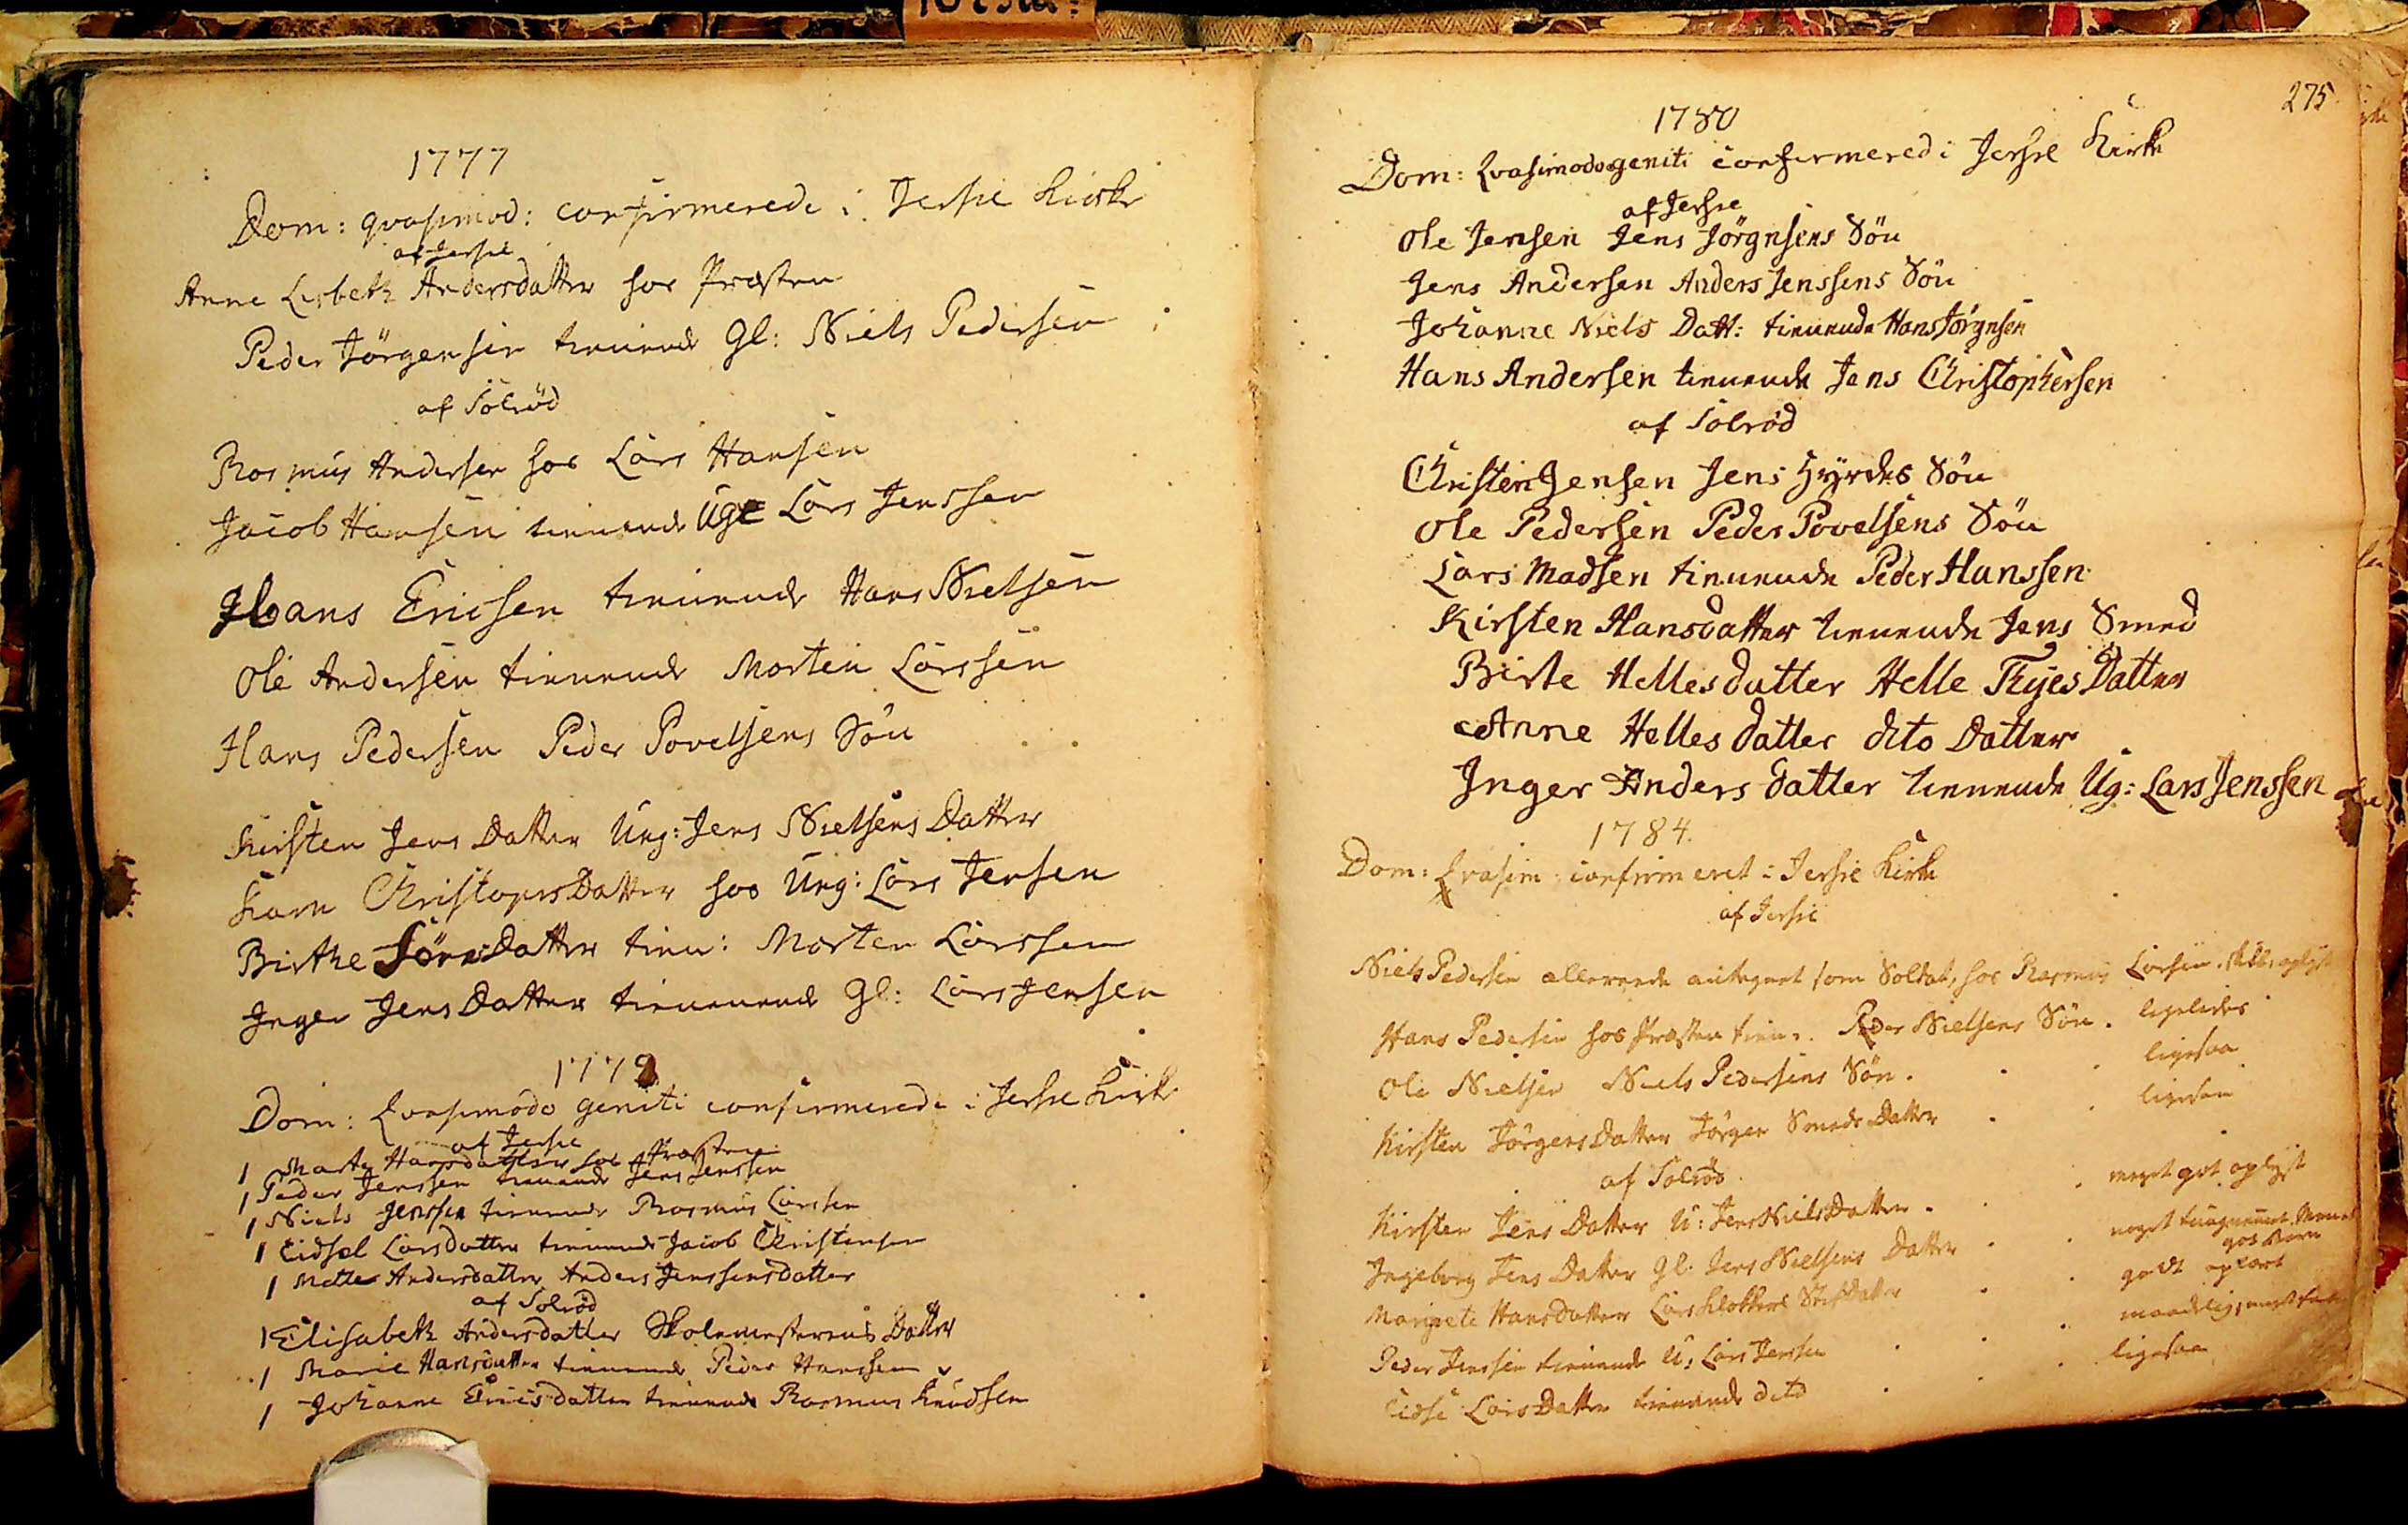1777
Dom: Qvasimod: Confirmerede i Jersie Kirke
af Jersie
Anne Lisbeth Andersdatter hos Præsten
Peder Jørgensen tiennende Gl: Niels Pedersen.
af Solrød
Rasmus Andersen hos Lars Hansen
Jacob Hansen tienende Ug Lars Jenssen
Hans Ericsen tienende Hans Nielsen
Ole Andersen tienende Morten Larssen
Hans Pedersen Peder Povelsens Søn
Kirsten Jens Datter Ung: Jens Nielsens Datter
Karn ChristophersDatter hos Ung: Lars Jensen
Birthe Sørns Datter tien: Morten Larssen
Inger Jens Datter tienende Gl: Lars Jensen
1779
Dom: Qvasimodo Geniti confirmerede i Jersie Kirke
af Jersie
Marte Hansdatter hos Præsten
Peder Jenssen tienende Jens Jenssen
Niels Jenssen tienende Rasmus Larssen
Cidsel Larsdatter tienende Jacob Christensen
Mette Andersdatter Anders Jenssens datter
af Solrød
Elisabeth Andersdatter Skolemesterens Datter
Marie Hansdatter tienende Peder Hanssen
Johanne Ericsdatter tienende Rasmus Knudsen
275.
1780
Dom: Qvasimodogeniti Confirmerede i Jersie Kirke
af Jersie
Ole Jensen Jens Jørgnsens Søn
Jens Andersen Anders Jenssens Søn
Johanne Niels Datt: tienende Hans Jørgnsen
Hans Andersen tienende Jens Christophersen
af Solrød
Christen Jensen Jens Hyrdes Søn
Ole Pedersen Peder Povelsens Søn
Lars Madsen tienende Peder Hanssen
Kirsten Hansdatter tienende Jens Smed
Birte Hellesdatter Helle Thyes Datter
Anne Hellesdatter dito Datter
Inger Anders datter tienende Ug: Lars Jenssen.
1784.
Dom: Qvasim: Confirmeret i Jersie Kirke
af Jersie
Niels Pedersen allerede antaget som Soldat, hos Rasmus Larsen. skikk: oplyst
Hans Pedersen hos Præsten tien: Peder Nielsens Søn ligeledes
Ole Nielsen Niels Pedersens Søn . . . ligesaa
Kirsten Jørgens Datter Jørgen Smeds Datter . . ligesaa
af Solrød
Kirsten Jens Datter U: Jens Niels Datter . . meget got oplyst
Ingeborg Jens Datter Gl: Jens Nielsens Datter . . noget tungnemmet. Men et
got Barn
Margrete Hans Datter Lars Klokkers Stifdatter . . godt oplært
Peder Jenssen tienende U: Lars Jenssen . . . maadelig; ## ##
Cidse Lars Datter tienende dito . . . ligesaa
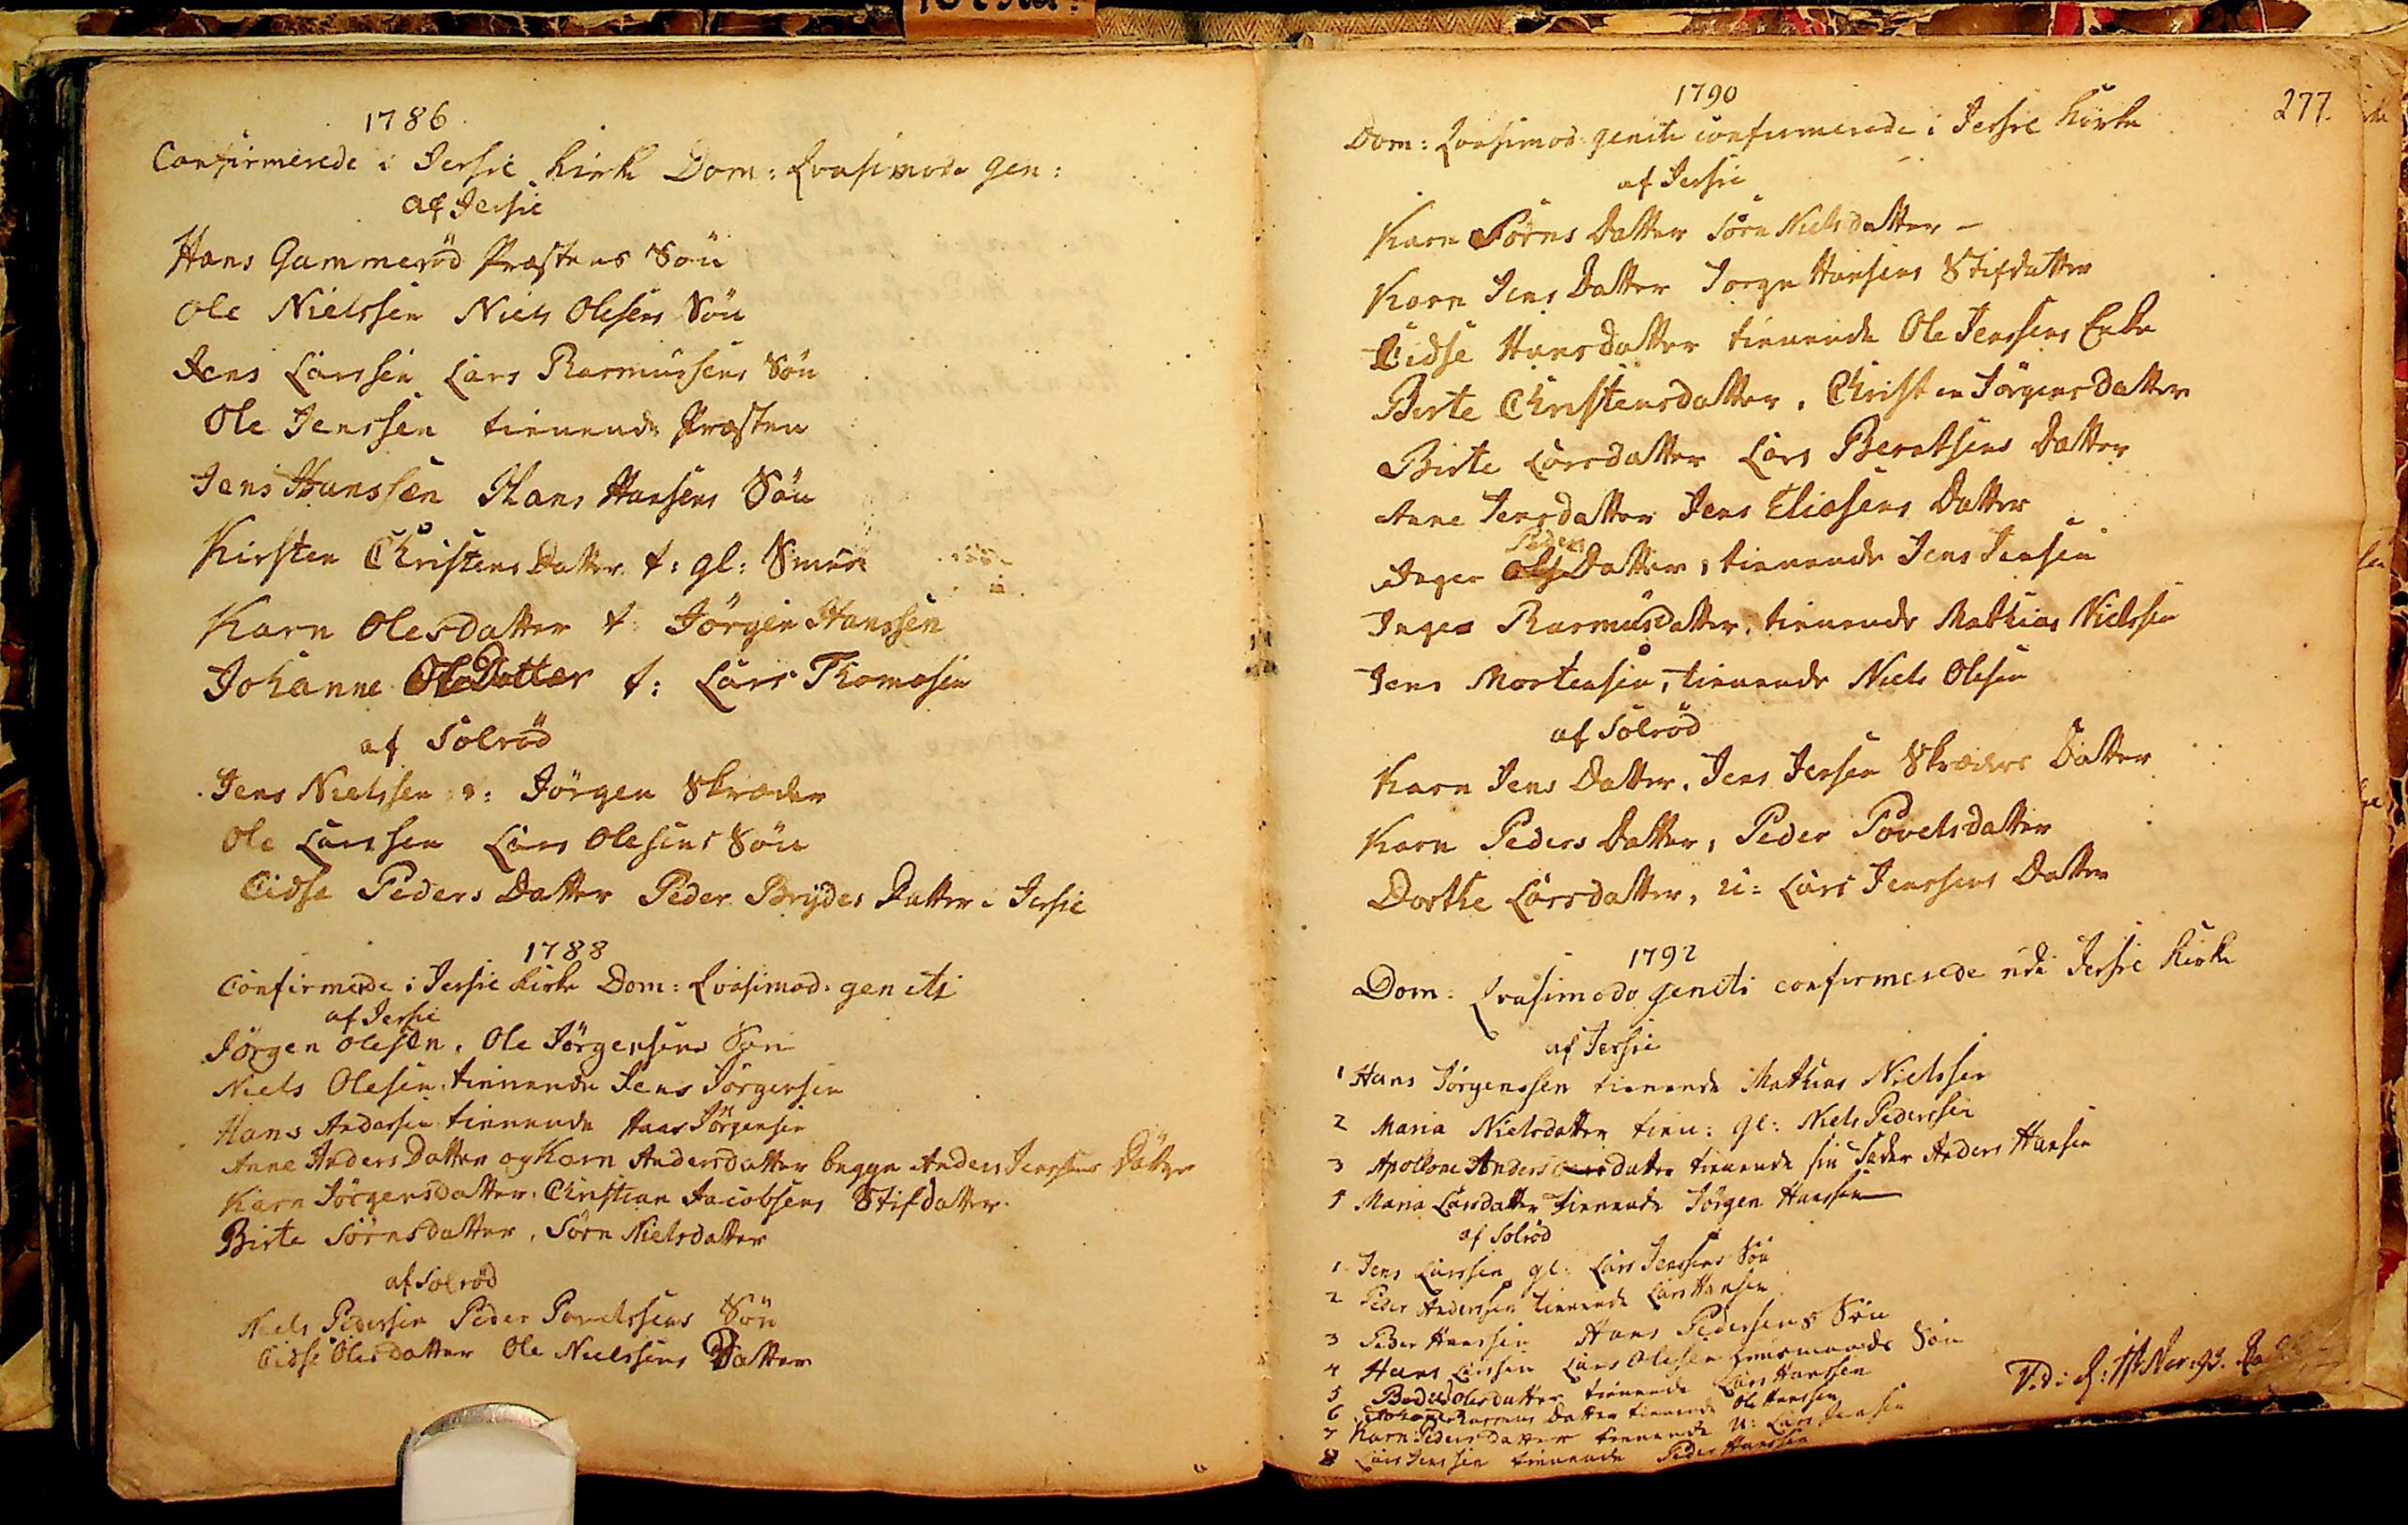1786
Confirmerede i Jersie Kirke Dom: Qvasimodo Gen:
af Jersie
Hans Gammerød Præstens Søn
Ole Nielssen Niels Olesens søn
Jens Larssen Lars Rasmussens Søn
Ole Jenssen tienende Præsten
Jens Hanssen Hans Hansens Søn
Kirsten Christens Datter t: gl: Smed
Karn Oles Datter t: Jørgen Hanssen
Johanne OleDatter t: Lars Thomæsen
af Solrød
Jens Nielssen t: Jørgen Skræder
Ole Larssen Lars Olesens Søn
Cidse Peders Datter Peder Brydes Datter i Jersie
1788
Confirmerede i Jersie Kirke Dom: Qvasimod: Geniti
af Jersie
Jørgen Olesen, Ole Jørgensens Søn
Niels Olesen, tienende Jens Jørgensen
Hans Andersen, tienende Hans Jørgensen
Anne Anders Datter og karn Andersdatter begge Anders Jenssens Døttre
Karn Jørgensdatter, Christian Jacobsens Stifdatter
Birte Sørnsdatter, Sørn Nielsdatter
af Solrød
Niels Pedersen Peder Povelsens Søn
Cidse Olesdatter Ole Nielssens Datter
277.
1790
Dom: Qvasimod: Geniti Confirmerede i Jersie Kirke
af Jersie
Karn Sørns Datter Sørn Niels Datter -
Karn Jens Datter Jørgn Hansens Stifdatter
Cidse Hansdatter tienende Ole Jenssens Enke
Birte Christensdatter. Christen Jørgens datter
Birte Larsdatter Lars Berntsens Datter
Anne Jensdatter Jens Eliasens Datter
Peders
Inger ##Datter, tienende Jens Jensen
Inge Rasmusdatter, tienende Mathias Nielssen
Jens Mortensen, tienende Niels Olesen
af Solrød
Karn Jens Datter. Jens Jensen Skræders Datter
Karn Peders Datter, Peder Povels datter
Dorthe Larsdatter, u: Lars Jenssens Datter
1792
Dom: Qvasimodo Geniti confirmerede udi Jersie Kirke
af Jersie
1 Hans Jørgenssen tienende Mathias Nielssen
2 Maria Nielsdatter tien: Gl: Niels Pederssen
3 Apollone Andersdatter tienende sin Fader Anders Hansen
4 Maria Larsdatter tienende Jørgen Hanssen __
af Solrød
1 Jens Larssen gl: Lars Jenssens Søn
2 Peder Anderssen tienende Lars Hansen
3 Peder Hanssen Hans Pedersens Søn
4 Hans Larssen Lars Olesen Huusmands Søn
5 Bodild Olesdatter tienende Lars Hanssen
6 Johane Rasmus Datter tienende Ole Hanssen
7 Karn Peders Datter tienende U: Lars Jensen
8 Lars Jenssen tienende Peder Hanssen
Vidi d: 1ste Nov:93. Rach##